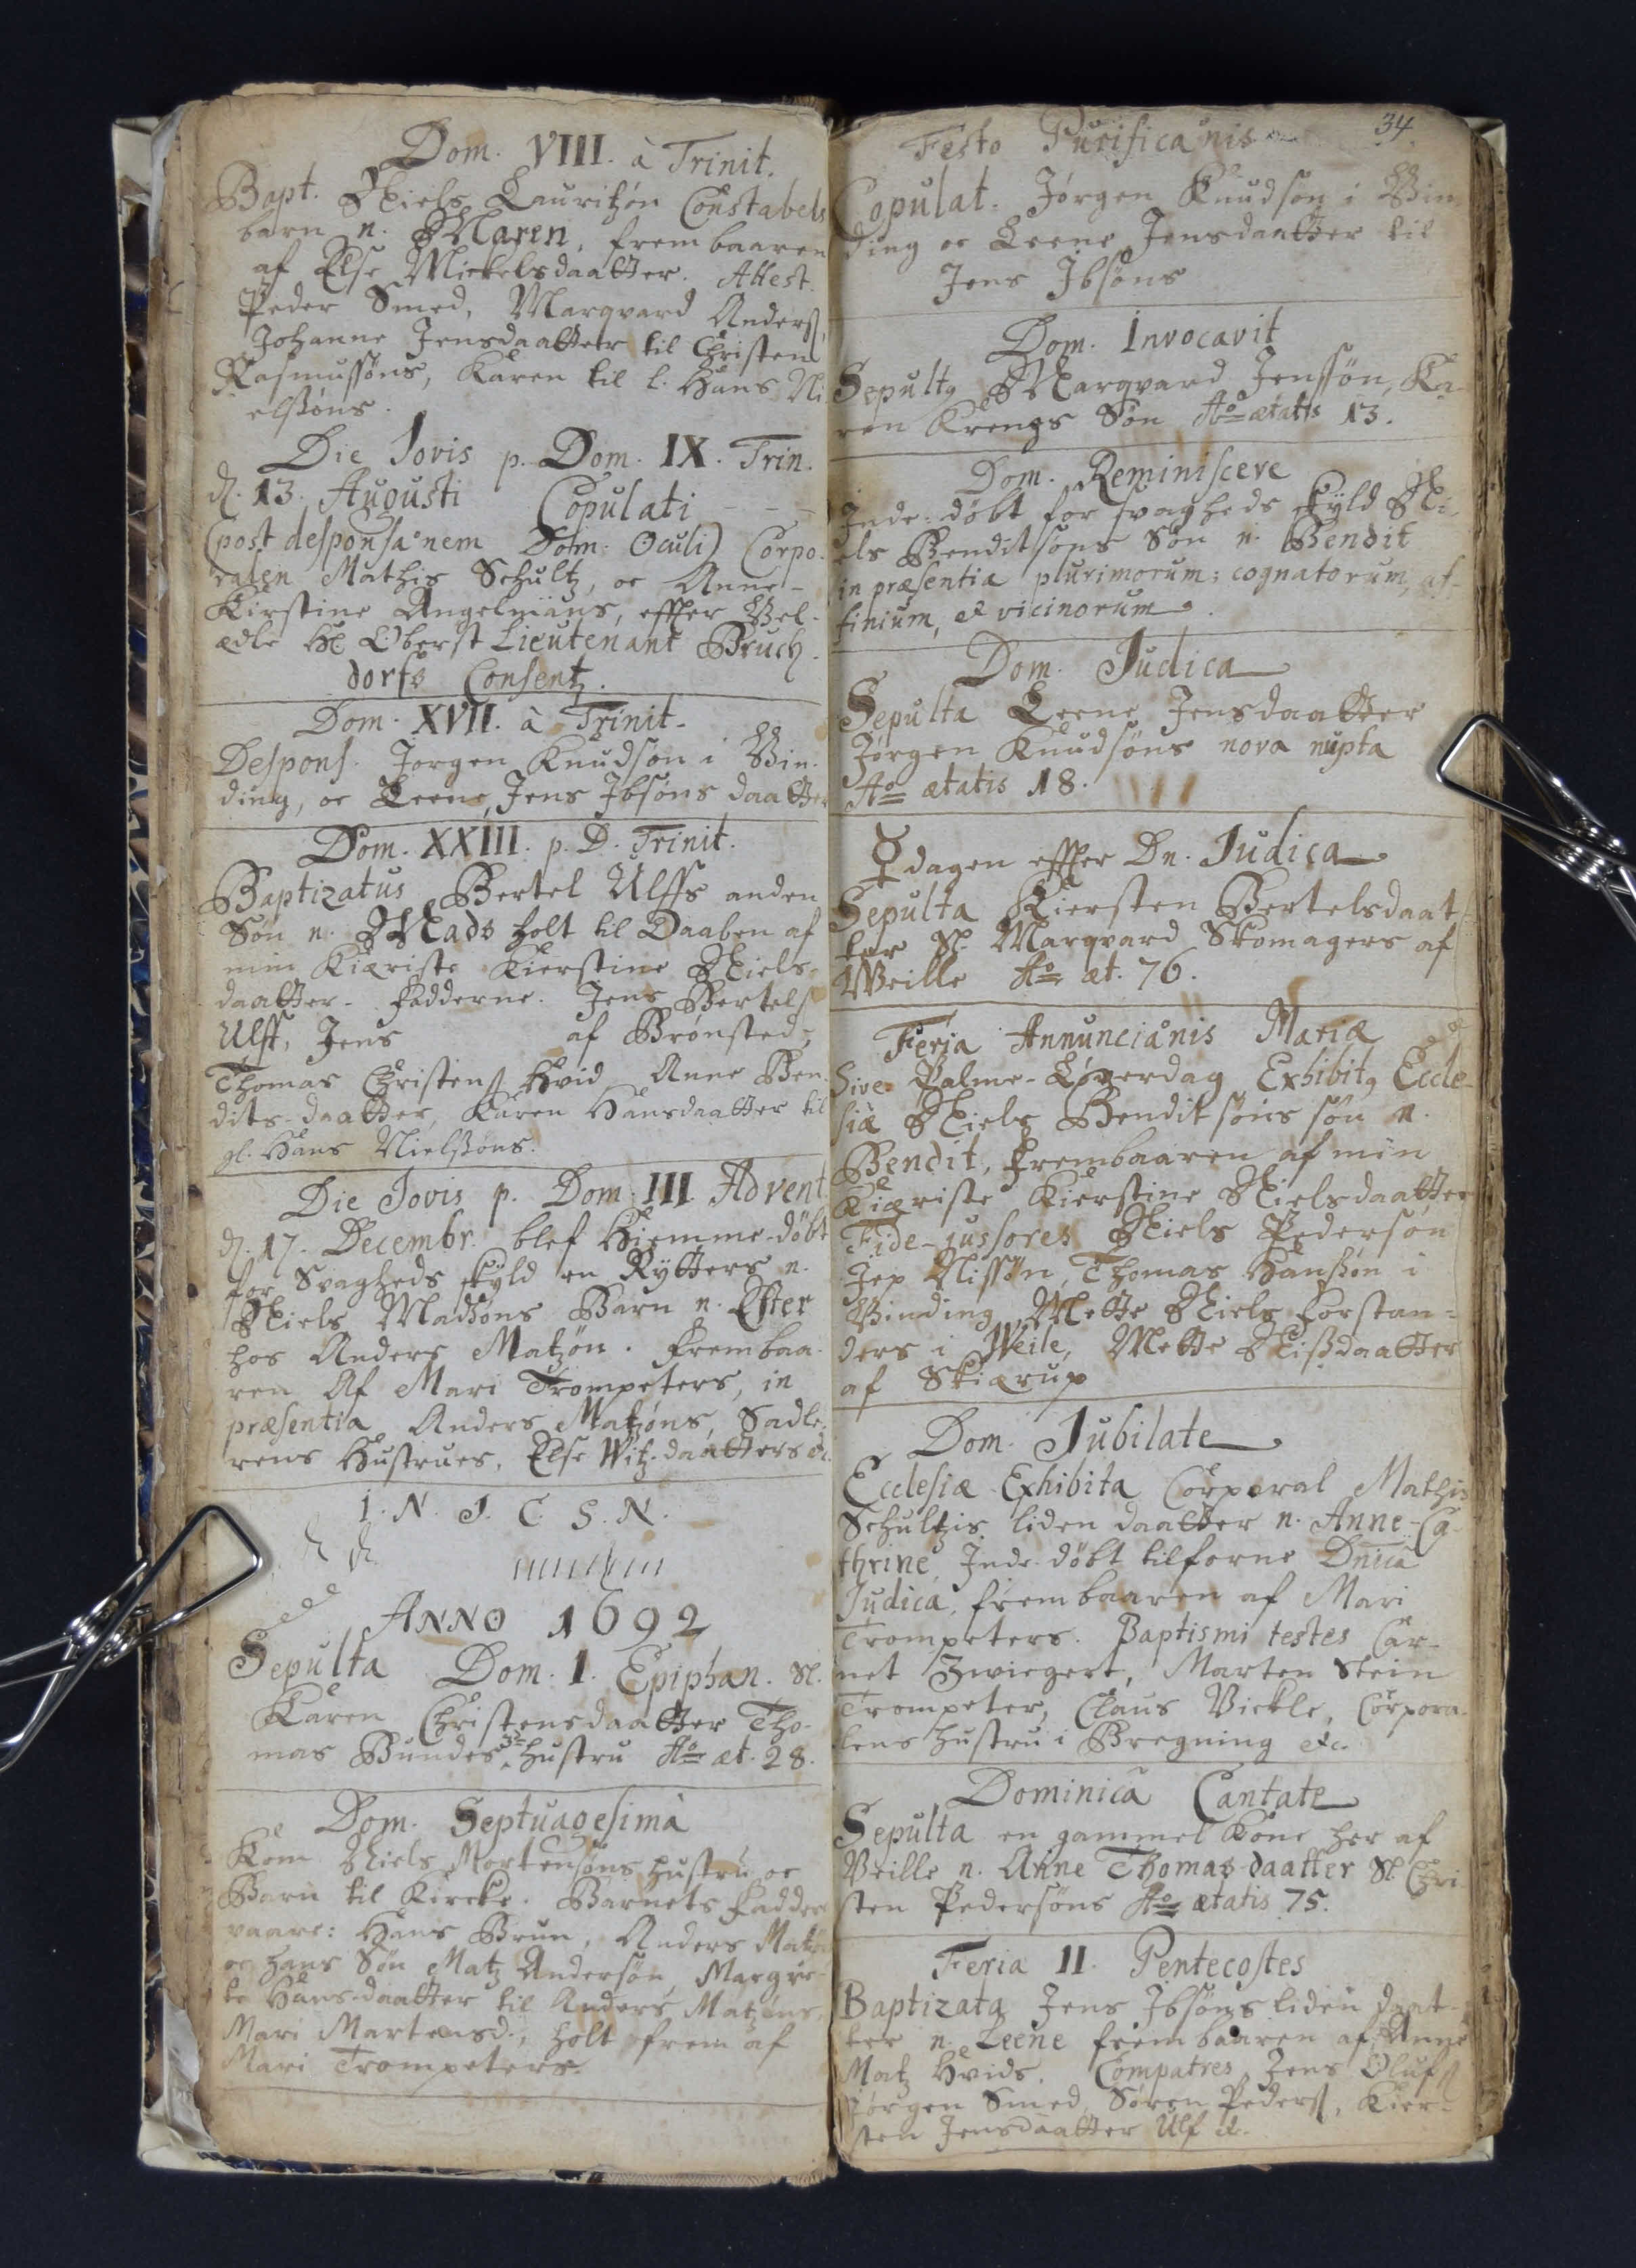Dom. VIII. a Trinit.
Bapt. Niels Lauritzøn Constabels 
barn n. Maren, frembaaren
af Else Mickelsdaatter. Attest
Peder Smed, Marqvard Anders
Johanne Jensdaatter til Christen 
Rasmussøns, Karen til l. Hans Ni
elssøns.
Die Jovis p. Dom. IX. Trin.
d. 13. Augusti.
Copulati
(post desponsanem Dom. Ovuli) Corpo.
ralen Mathis Schultz oc Anne
Kirstine Angelmans, effter Vel¬
ædle Hl Oberst Lieutenant Bruch¬
dorfs Consentz.
Dom. XVII a Trinit.
Despons Jørgen Knudsøn i Vin
ding, oc Leene, Jens Ibsøns daatter
Dom. XXIII p. D. Trinit.
Baptizatus Bertel Ulffs anden
Søn n. MADS, holt til Daaben af 
min Kiæriste Kierstine Niels
daatter. Fadderne. Jens Bertels
Ulff, Jens
af Brønsted
Thomas Christens Hvid Anne Ben
dits-daatter, Karen Hansdaatter til
gl. Hans Nielssøns.
Die Jovis p. Dom. III Advent
d. 17. Decembr. blef hieme døbt
for Svagheds skyld en Rytters n.
Niels Madtzøns Barn n. Ester
hos Anders Madtzøn. frembaa¬
ren Af Mari Trompeters, in
præsentia Anders Matzøns, Sadle
rens Hustrues, Else Witzdaatters etc.
I. N. J. C. S. N.
Anno 1692
Sepulta Dom. 1 Epiphan. Sl.
Karen Christensdaatter Tho¬
3d
mas Bundes hustru Ao æt. 28.
Dom. Septuagesima
Kom Niels Mortensøns hustru oc
Barn til Kircke. Barnets faddere
vaare: Hans Brun, Anders Matzøn
oc hans Søn Matz Andersøn, Margre¬
te Hansdaatter til Anders Matzøns
Mari Martensd., holt frem af
Mari Trompeters.
34
Festo Purificanis
Copulat. Jørgen Knudsøn i Vin
ding oc Leene Jensdaatter til 
Jens Ibsøns
Dom. Invocavit
Sepultq Marqvard Jenssøn, Ka
ren Krengs Søn Ao ætatis 13.
Dom. Reminiscere
Inde=døbt for svagheds skyld Ni¬
els Benditsøns Søn n. Bendit
in præsenti purimorum; cognatorum, af
finium, et vicinorum
Dom. Judica
Sepulta. Leene Jensdaatter
Jørgen Knudsøns nova nupta
Ao ætatis 18
☿ dagen effter Dn. Judica
Sepulta Kiersten Bertelsdaat
ter Sl. Marqvard Skomagers af
Weille Ao æt. 76.
Feria Annuncianis Mariæ
Sive Palme-Løverdag Exhibit Eccle
siæ Niels Benditsøns søn n.
Bendit, frembaaren af min
Kiæriste Kierstine Nielsdaatter,
Fide-jussores Niels Pedersøn.
Jep Nielsøn, Thomas Hansøn i
Vinding. Mette Niels Forstan
ders i Weile, Mette Nissdaatter
af Skiærup
Dom. Jubilate
Ecclesiæ Exhibita Corporal Mathis
Schultzis liden daatter n. Anne-Ca
thrine Inde døbt tilforne Dnica
Judica, frembaaren af Mari
Trompeters. Baptismi testes. Cor
net Zcviegert##, Morten Stein
Trompeter, Claus Vickle, Corpora¬
lens hustru i Bregning etc.
Dominica Cantate
Sepulta en gammel Kone her af
Veille n. Anne Thomas daatter Sl Chri¬
sten Pedersøns Ao ætatis 75.
Feria II Pentecostes
Baptizata Jens Ibsøns liden daat¬
ter n. Leene, frem baaren af Anne
Matz Hvids. Compatres Jens Olufs
Jørgen Smed, Søren Peders Kier¬
sten Jensdaatter Ulf etc.
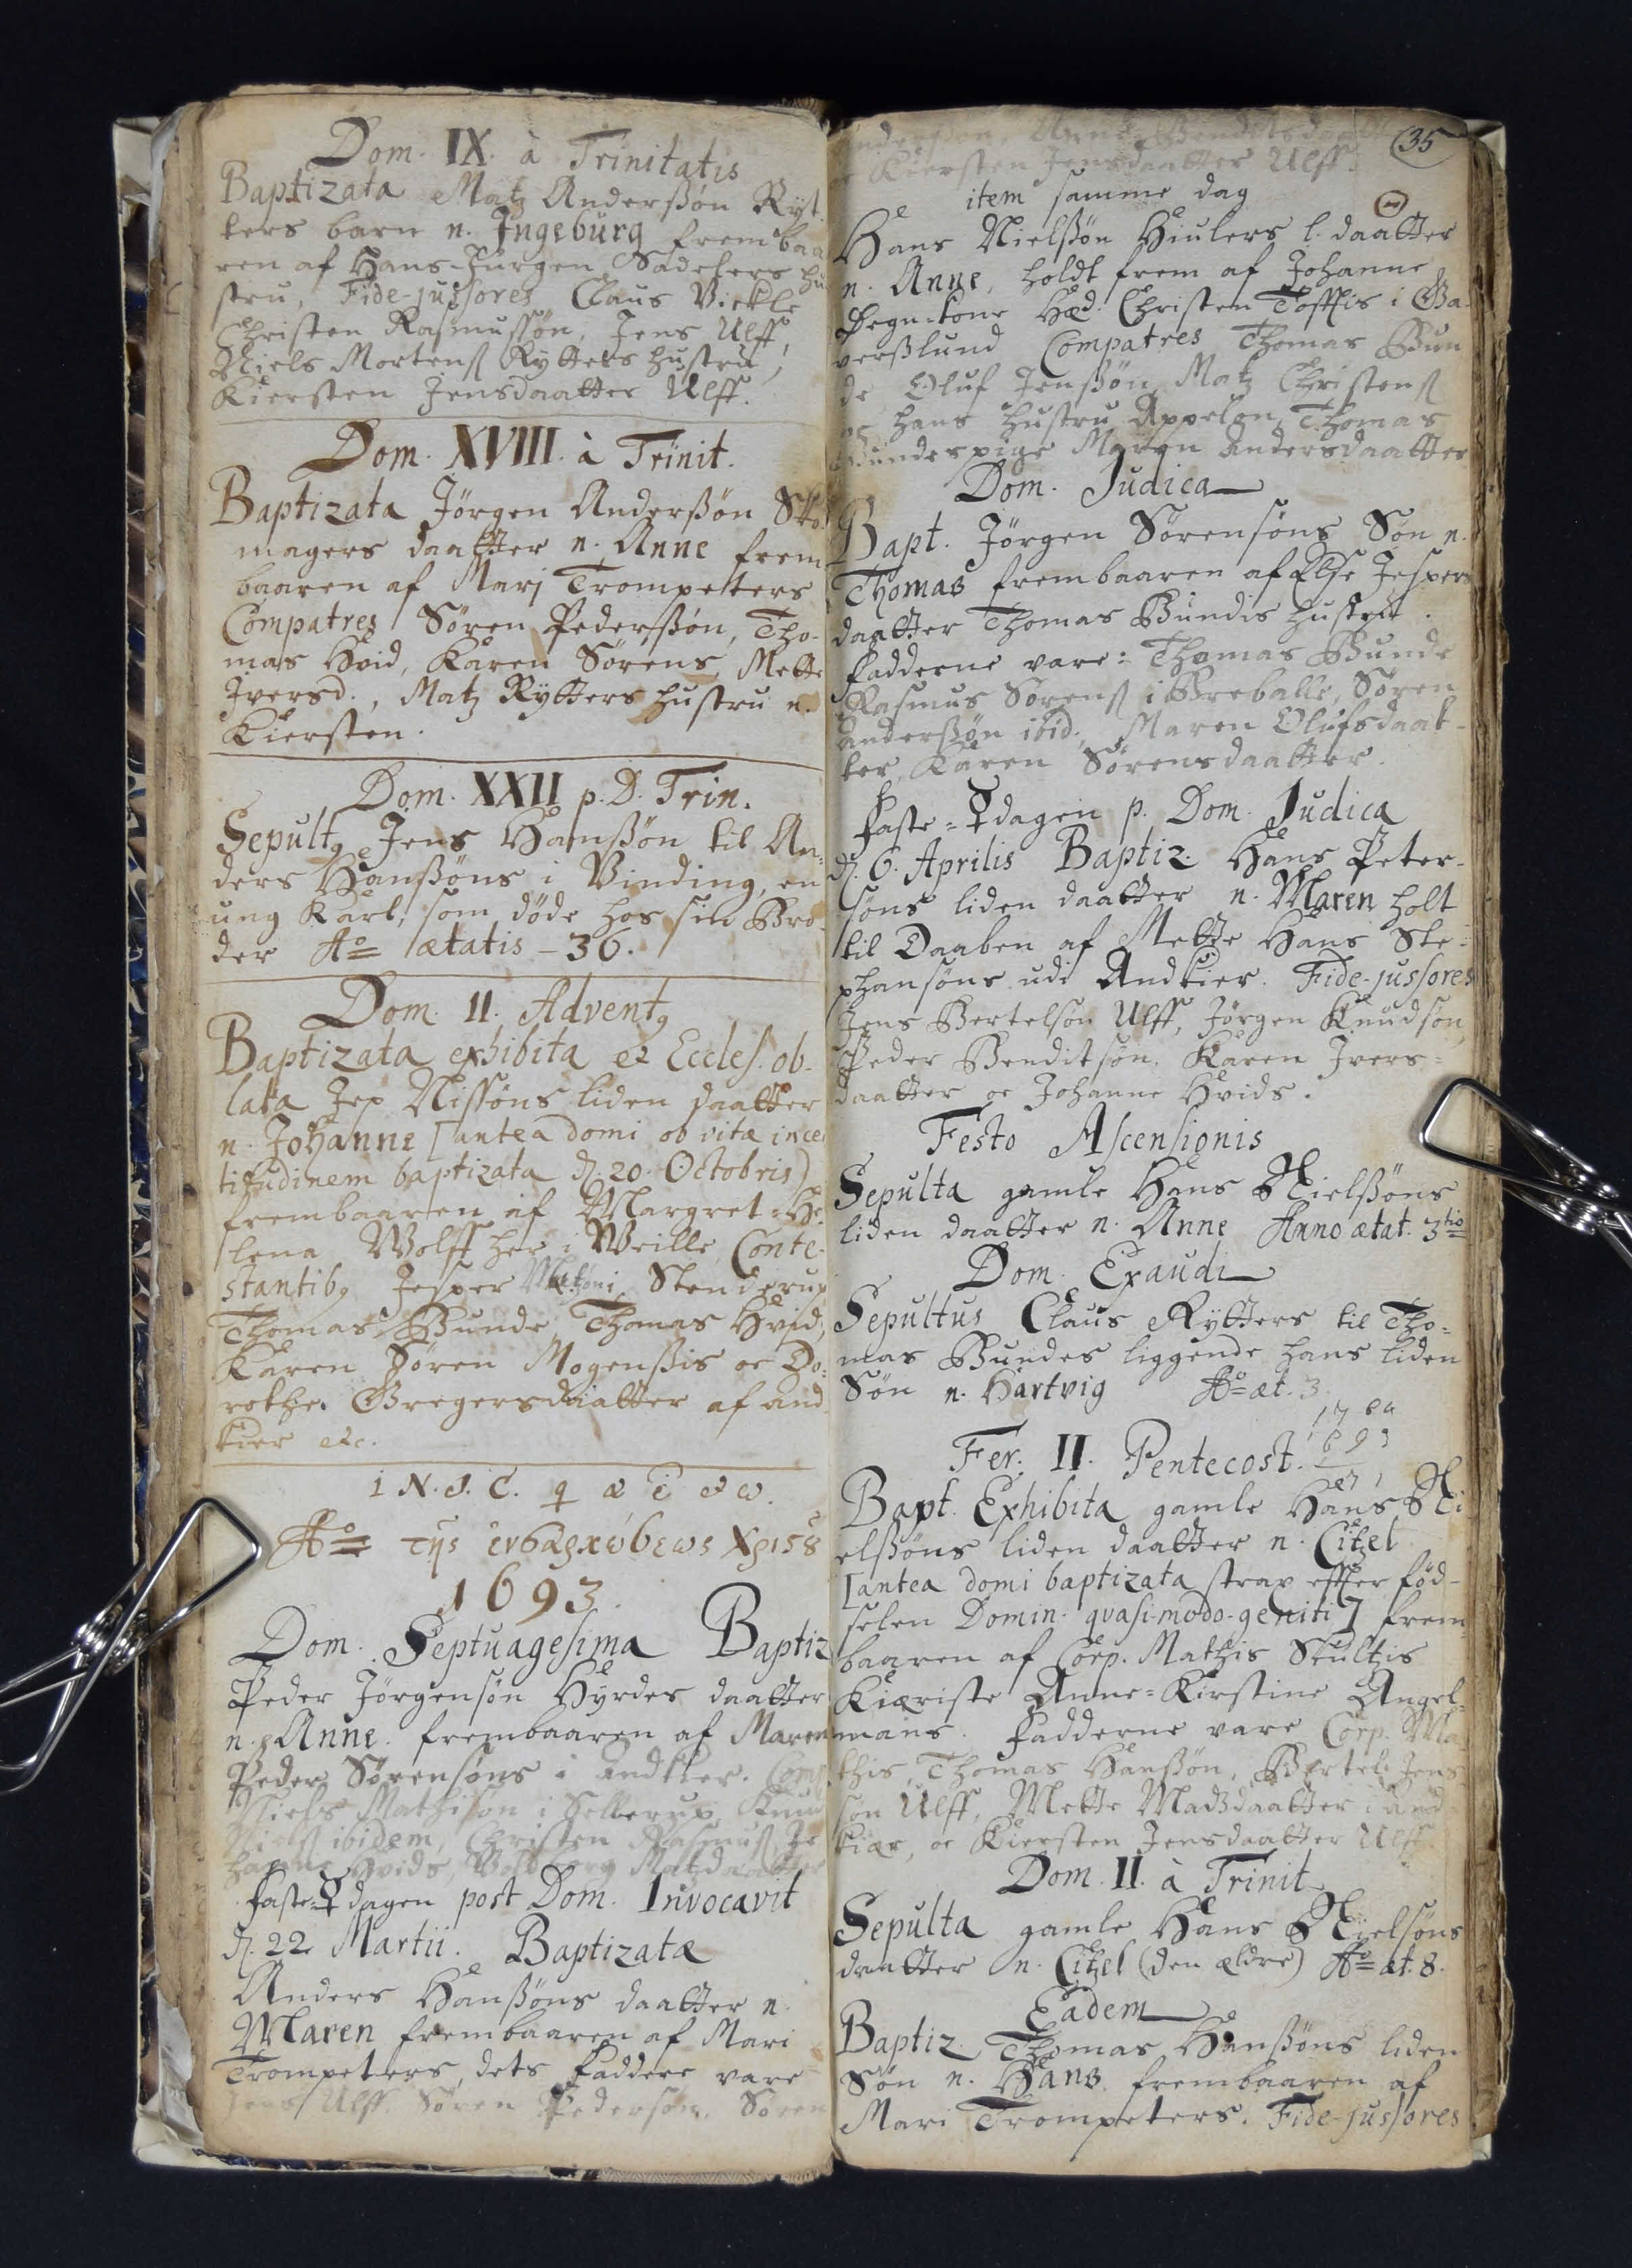Dom. IX a Trinitatis
Baptizata Matz Anderssøn Ryt¬
ters barn n. Ingeburg frembaa
ren af Hans Jurgen Sadelers hu
atru,. Fide-jussores Claus Vickle
Christen Rasmussøn, Jens Ulff
Niels Mortens Rytters hustru
Kiersten Jensdaatter Ulff
Dom. XVIII a Trinit
Baptizata Jørgen Anderssøn Sko
magers daatter n. Anne frem
baaren af Marj Trompeters
Compatres Søren Pederssøn, Tho¬
mas Hvid, Karen Sørens, Mette
Iversd., Matz Rytters hustru n.
Kiersten
Dom. XXII p. D. Trin.
Sepultq Jens Hanssøn til An¬
ders Hanssøns i Vinding, en
ung karl, som døde hos sin Bro¬
der Ao ætatis 36.
Dom. II Adventq
Baptizata exhibita et Eccles ob
lata Jep Nissøns liden daatter
n. Johanne [antea domi ob vitæ incer
ti judinem baptizata d. 20 Octobris) 
frembaaren af Margrete = He¬
lena Wolff her i Weille Conte¬
stantibq Jesper Matzøn i Stenderup
Thomas Bunde Thomas Hvid;
Karen Søren Mogenssis oc Do¬
rthe Gregersdaatter af And
kier etc
I. N. J. C. q a ## et ##
Ao Tys## cvbalx##be##s Xpi58
1693
Dom. Septuagesima Baptiz
Peder Jørgensøn Hyrdes daatter 
n. Anne frembaaren af Maren
Peder Sørensøns i Andkier. Comp
Niels Mathisøn i Sellerup. Knud
Niels ibidem, Christen Rasmus Jo
hanne Hvids,. Woldborg Matzdaatter
Faste ☿ dagen post Dom. Invocavit
d. 22 Martii. Baptizata 
Anders Hanssøns daatter n.
Maren, frembaaren af Mari 
Trompeters, dets faddere vare 
Jens Ulf. Søren Pedersøn. Søren
35
Anderssøn##, ## Binditsdaatter##
oc Kiersten Jensdaatter Ulf.
item samme dag
Hans Nielssøn Hiulers l. daatter
n. Anne, holdt frem af Johanne 
Degn-kone Hæd. Christen Tofftis i Ga
versslund Compatres Thomas Bun
de Oluf Jenssøn. Matz Christens
oc hans hustru Appelon, Thomas
Bundes pige Maren Andersdaatter.
Dom. Judica
Bapt. Jørgen Sørensøns Søn n. 
Thomas frembaaren af Else Jespers
daatter Thomas Bundis hustru
Fadderne vare: Thomas Bunde
Rasmus Sørens i Breballe, Søren
Anderssøn ibid. Maren Olufsdaat¬
ter,. Karen Sørensdaatter
Faste = ☿ dagen p. Dom. Judica
d. 6. Aprilis Baptiz. Hans Peter
søns liden daatter n. Maren, holdt 
til Daaben af Mette Hans Ste¬
phansøns udi Andkier. Fide-jussores. 
Jens Bertelsøn Ulff, Jørgen Knudsøn
Peder Benditsøn. Karen Ivers¬
daatter oc Johanne Hvids.
Festo Ascensionis
Sepulta gamle Hans Nielssøns
liden daatter n. Anne, Anno ætat. 3tio
Dom. Exaudi
Sepultus Claus Rytters til Tho¬
mas Bundes liggende hans liden 
Søn n. Hartvig, 
Ao æt. 3.
Fer. II Pentecost.
Bapt. Exhibita gamle Hans Ni
elsøns liden daatter n. Citzel 
[antea domi baptizata strax efter fød¬
selen Domin. qvasimodo-geniti] frem
baaren af Corp. Mathis Schultzis
Kiæriste Anne=Kirstine Angel¬
mans. Fadderne vare Corp. Ma
this, Thomas Hanssøn. Berthel Jens¬
søn Ulff, Mette Madzdaatter i And¬
kiær, oc Kiersten Jensdaatter Ulff.
Dom. II. a Trinit
Sepulta gamle Hans Nielsøns
daatter n. Citzel (den ældre) Ao æt. 8.
Eodem
Baptiz Thomas Hanssøns liden
Søn n. Hans, frembaaren af
Mari Trompeters. Fide-jussores